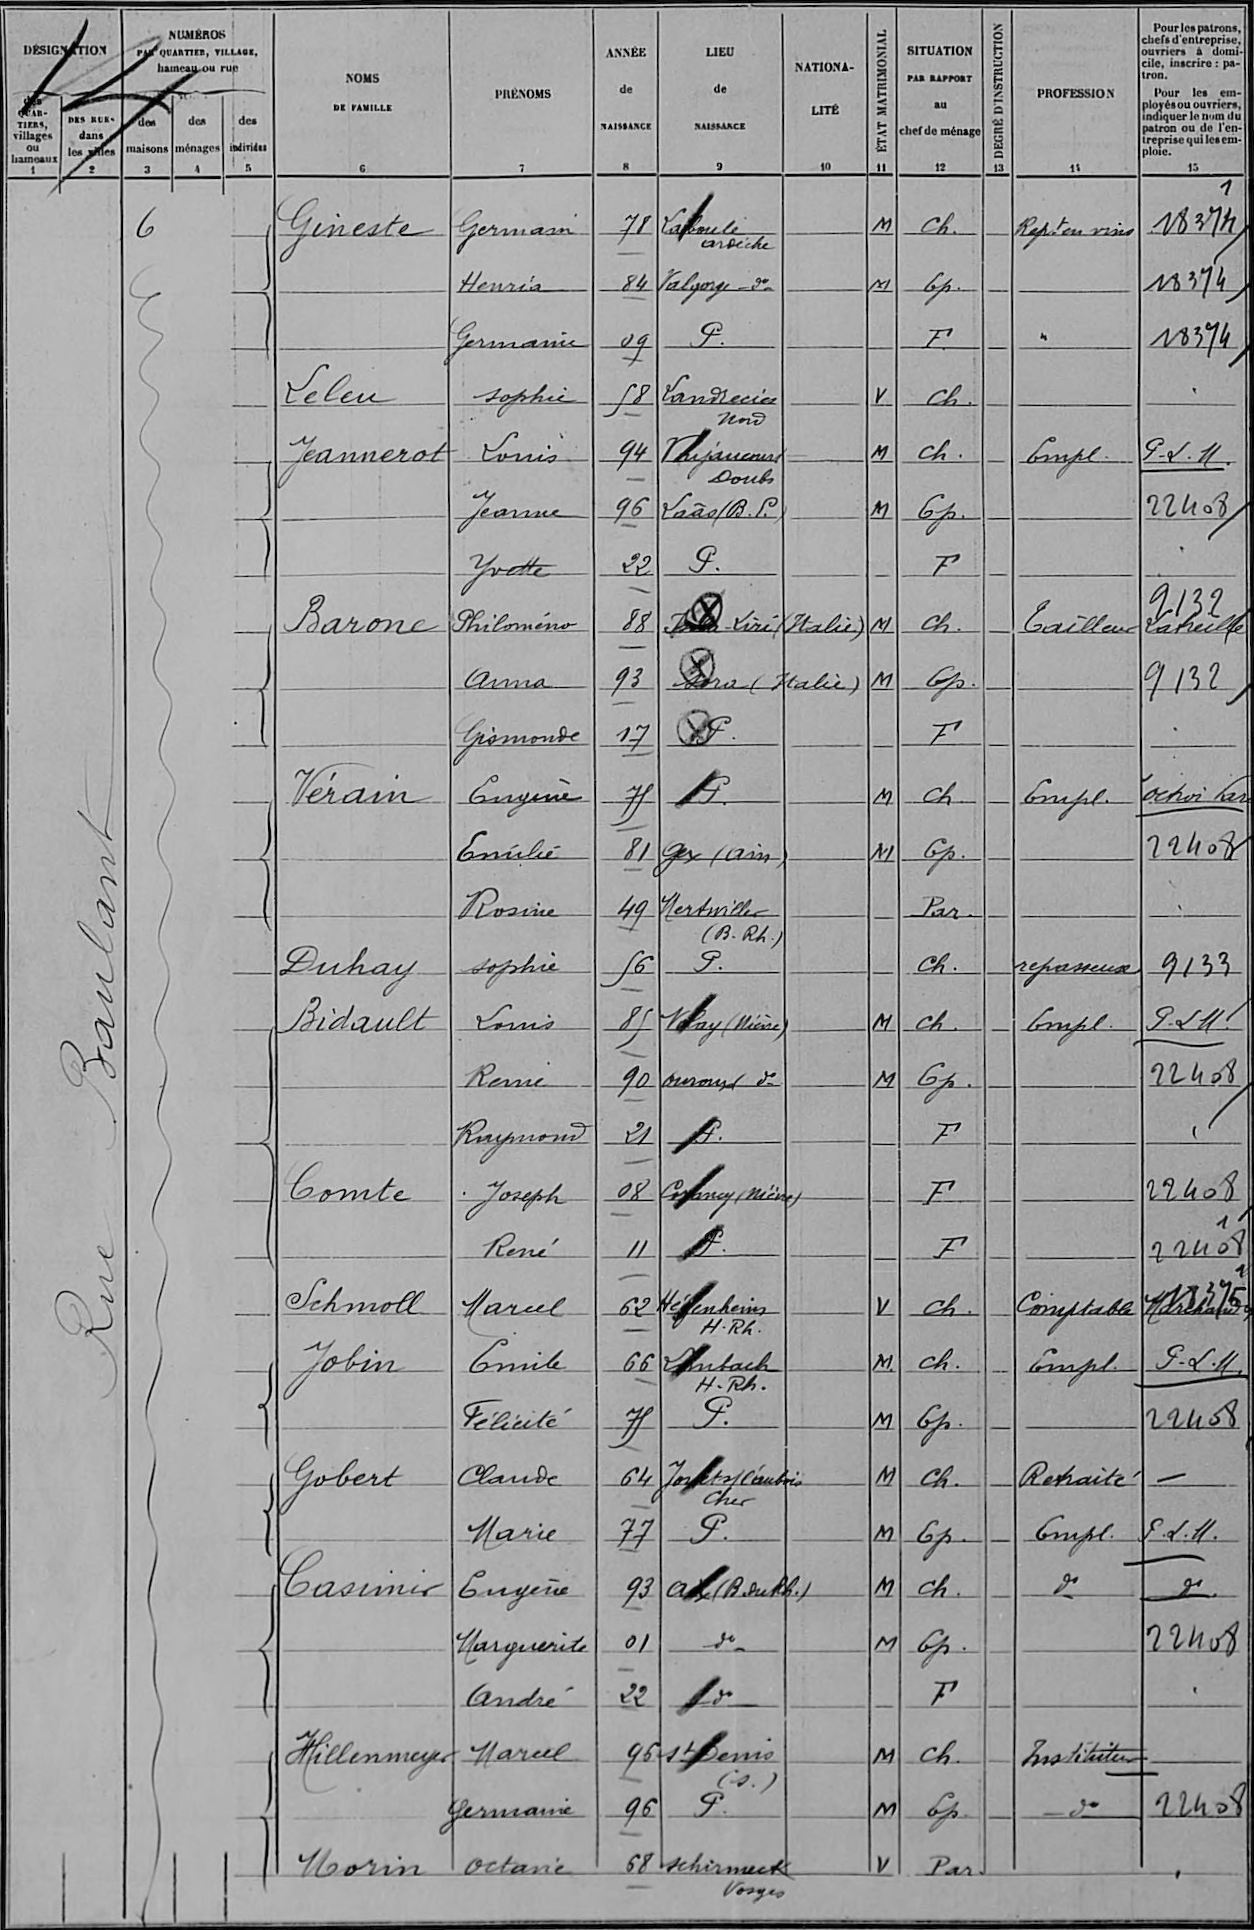Gineste/Germain/78/Laboule!ardèche/¤/M/Ch /¤/Rept en vins/18374
¤/Henria/84/Valgorge d°/¤/M/Ep /¤/¤/18374
¤/Germaine/09/P /¤/¤/F/¤/¤/18374
Leleu/sophie/58/Landrecies ! Nord/¤/V/Ch /¤/¤/¤
Jeannerot/Louis/94/Voujaucourt ! Doubs/¤/M/ch /¤/Empl/P L M
¤/Jeanne/96/Laàs (B P )/¤/M/Ep /¤/¤/22408
¤/Yvette/22/P /¤/¤/F/¤/¤/¤
Barone/Philoméno/88/Isola Liri (Italie)/¤/M/Ch /¤/Tailleur/Latreille?9132
¤/Anna/93/Sora (Italie)/¤/M/Ep /¤/¤/9132
¤/Gismonde/17/P /¤/¤/F/¤/¤/¤
Vérain/Eugène/75/P /¤/M/Ch/¤/Empl /octroi Paris
¤/Emilie/81/Gex (Ain)/¤/M/Ep /¤/¤/22408
¤/Rosine/49/Mertwiller!(B Rh )/¤/¤/Par /¤/¤/¤
Duhay/sophie/56/P /¤/¤/Ch /¤/repasseuse/9133
Bidault/Louis/85/Nolay (Nièvre)/¤/M/ch /¤/Empl/P L M
¤/René/90/Ouroux d°/¤/M/Ep /¤/¤/22408
¤/Raymond/21/P /¤/¤/F/¤/¤/¤
Comte/Joseph/08/Corancy (Nièvre)/¤/¤/F/¤/¤/22408
¤/René/11/P /¤/¤/F/¤/¤/22408
Schmoll/Marcel/62/Hégenheim!H Rh/¤/V/Ch /¤/Comptable/Marchand?18375
Jobin/Emile/66/Leimbach!H Rh /¤/M/ch /¤/Empl/PLM
¤/Félicité/75/P /¤/M/Ep /¤/¤/22408
Gobert/Claude/64/Jouet s: l'aubois!Cher/¤/M/Ch /¤/Retraité/¤
¤/Marie/77/P /¤/M/Ep /¤/Empl/P L M
Casimir/Eugène/93/Aix (B du Rh )/¤/M/ch /¤/d°/d°
¤/Marguerite/01/d°/¤/M/Ep /¤/¤/22408
¤/André/22/d°/¤/¤/F/¤/¤/¤
Hillenmeyer/Marcel/96/st Denis!(s )/¤/M/ch /¤/Instituteur/¤
¤/Germaine/96/P /¤/M/Ep /¤/d°/22408
Morin/Octavie/68/schirmeck!Vosges/¤/V/Par /¤/¤/¤
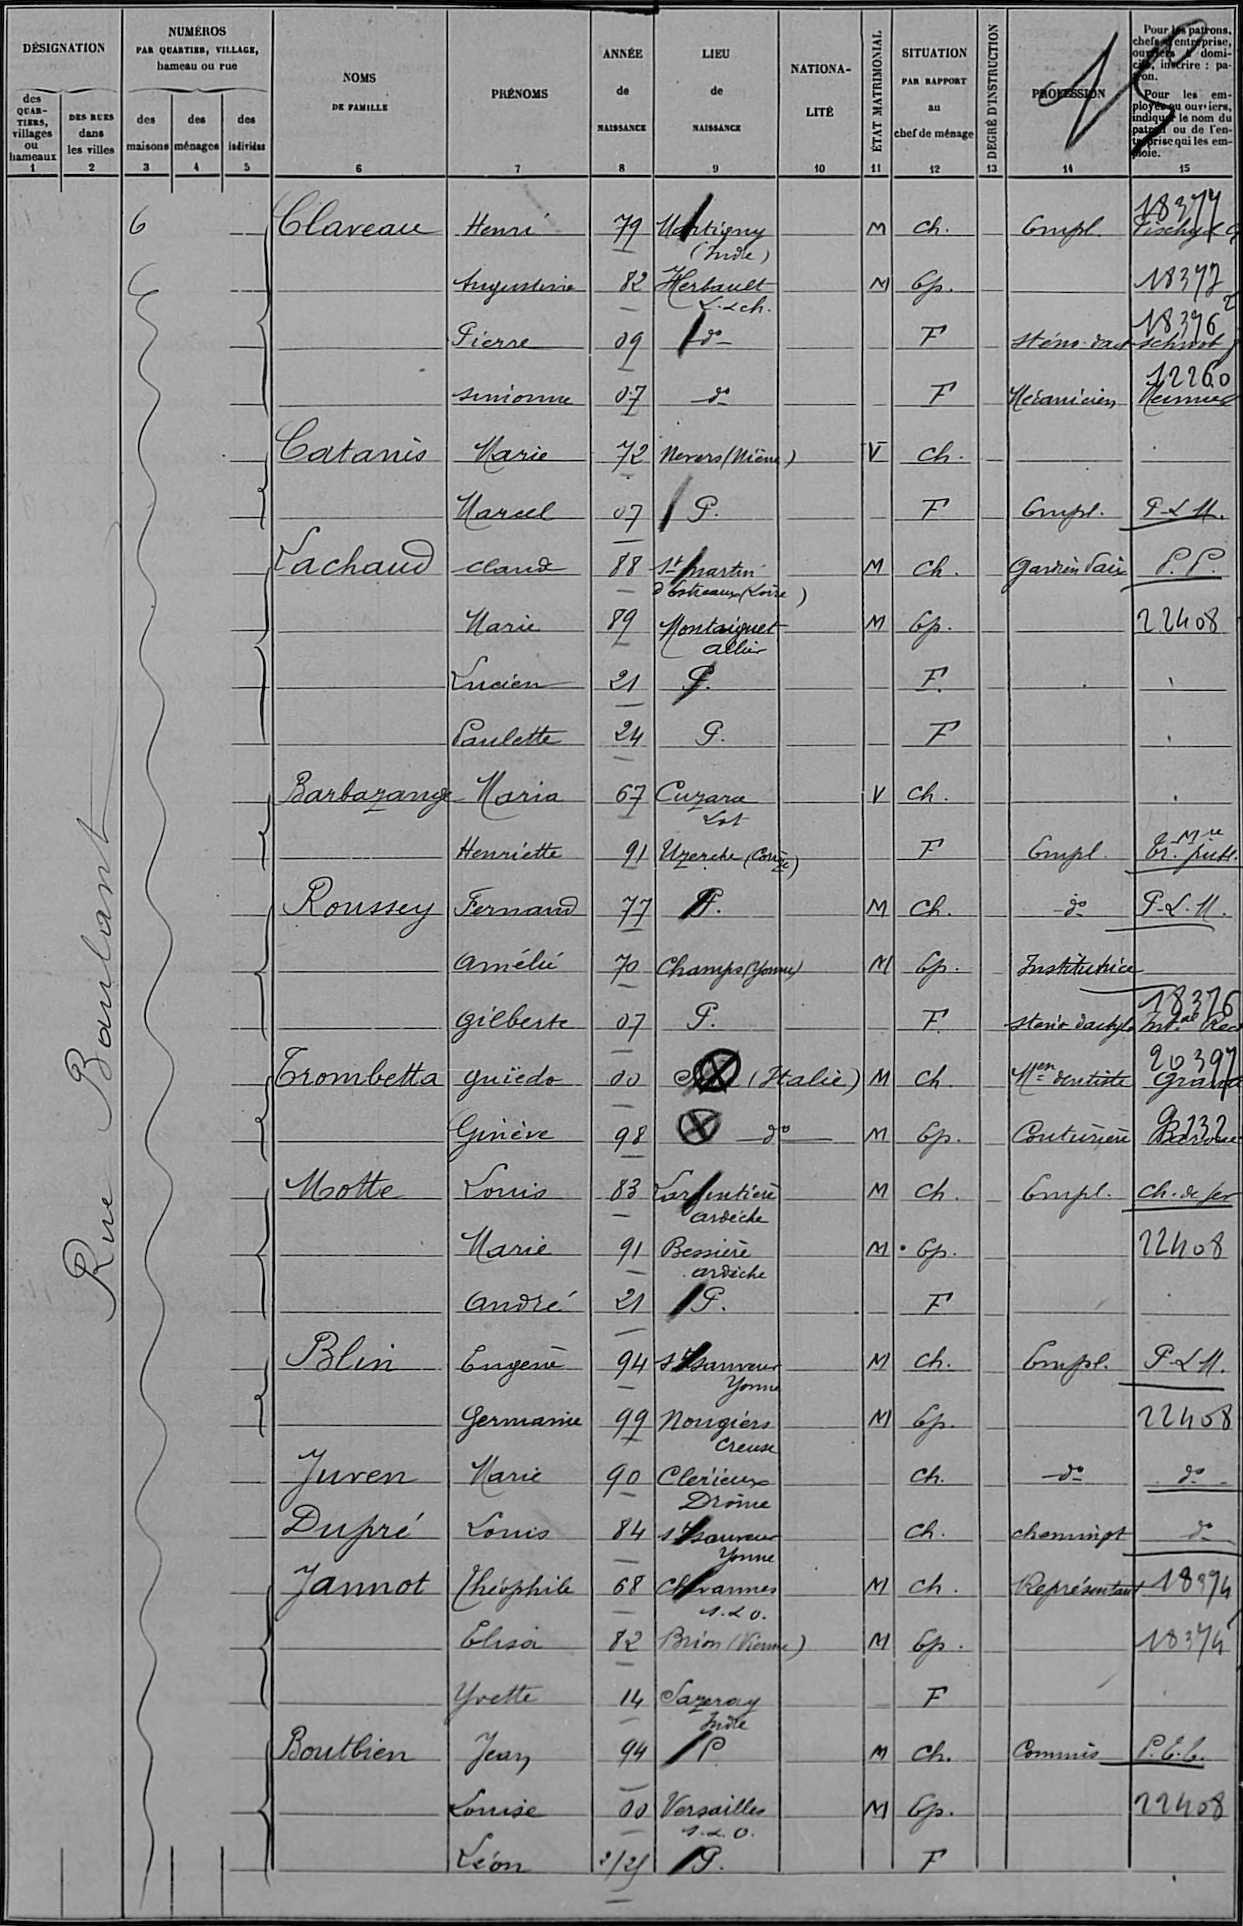Claveau/Henri/79/Martigny!(Indre)/¤/M/ch /¤/Empl /Fischyot ? 18377
¤/Augustine/82/Herbault!L&ch /¤/M/Ep /¤/¤/18377
¤/Pierre/09/d°/¤/¤/F/¤/sténo dact/schmob?18376
¤/Simonne/07/d°/¤/¤/F/¤/Mécanicien/Mermier?12260
Catanis/Marie/72/Nevers (Nièvre)/¤/V/ch /¤/¤/¤
¤/Marcel/07/P /¤/¤/F/¤/Empl/P L M
Lachaud/Claude/88/St Martin!d'Estreaux (Loire)/¤/M/ch /¤/gardien paix/P P
¤/Marie/89/Monteignet!allier/¤/M /Ep /¤/¤/22408
¤/Lucien/21/P /¤/¤/F /¤/¤/¤
¤/Paulette/24/P /¤/¤/F/¤/¤/¤
Barbarange/Maria/67/Cuzance!Lot/¤/V/ch /¤/¤/¤
¤/Henriette/91/Uzerche (Corrèze)/¤/¤/F/¤/Empl /Tr publ ? Mre
Roussey/Fernand/77/P /¤/M/Ch /¤/d°/P L M
¤/Amélie/70/Champs (Yonne)/¤/M/Ep /¤/Institutrice/¤
¤/gilberte/07/P /¤/¤/F /¤/steno dactylo/Int Rect?18376
Crombetta/guïedo/00/(Italie)/¤/M/ch /¤/Men dentiste/Grand?20397
¤/Ginève/98/d°/¤/M/Ep /¤/Couturière/Baroue?9132
Motte/Louis/83/Largentière ! ardèche/¤/M/ch /¤/Empl /ch de fer
¤/Marie/91/Bessière!ardèche/¤/M/Ep /¤/¤/22408
¤/André/21/P /¤/¤/F/¤/¤/¤
Blin/Eugène/94/St Sauveur!Yonne/¤/M/ch /¤/Empl /P L M
¤/Germaine/99/Nouziers!Creuse/¤/M/Ep /¤/¤/22408
Juven/Marie/90/Clérieux!Drôme/¤/¤/ch /¤/d°/d°
Dupré/Louis/84/St Sauveur ! Yonne/¤/¤/ch /¤/cheminot/d°
Jannot/Théophile/68/Chevannes!s & o /¤/M/ch /¤/Représentant/18374
¤/Elisa/82/Brion (Vienne)/¤/M/Ep /¤/¤/18374
¤/Yvette/14/Sazeray!Indre/¤/¤/F/¤/¤/¤
Boutbien/Jean/94/P/¤/M/ch /¤/Commis/P T T
¤/Louise/00/Versailles!S & O/¤/M/Ep /¤/¤/22408
¤/Léon/2:21/P /¤/¤/F/¤/¤/¤